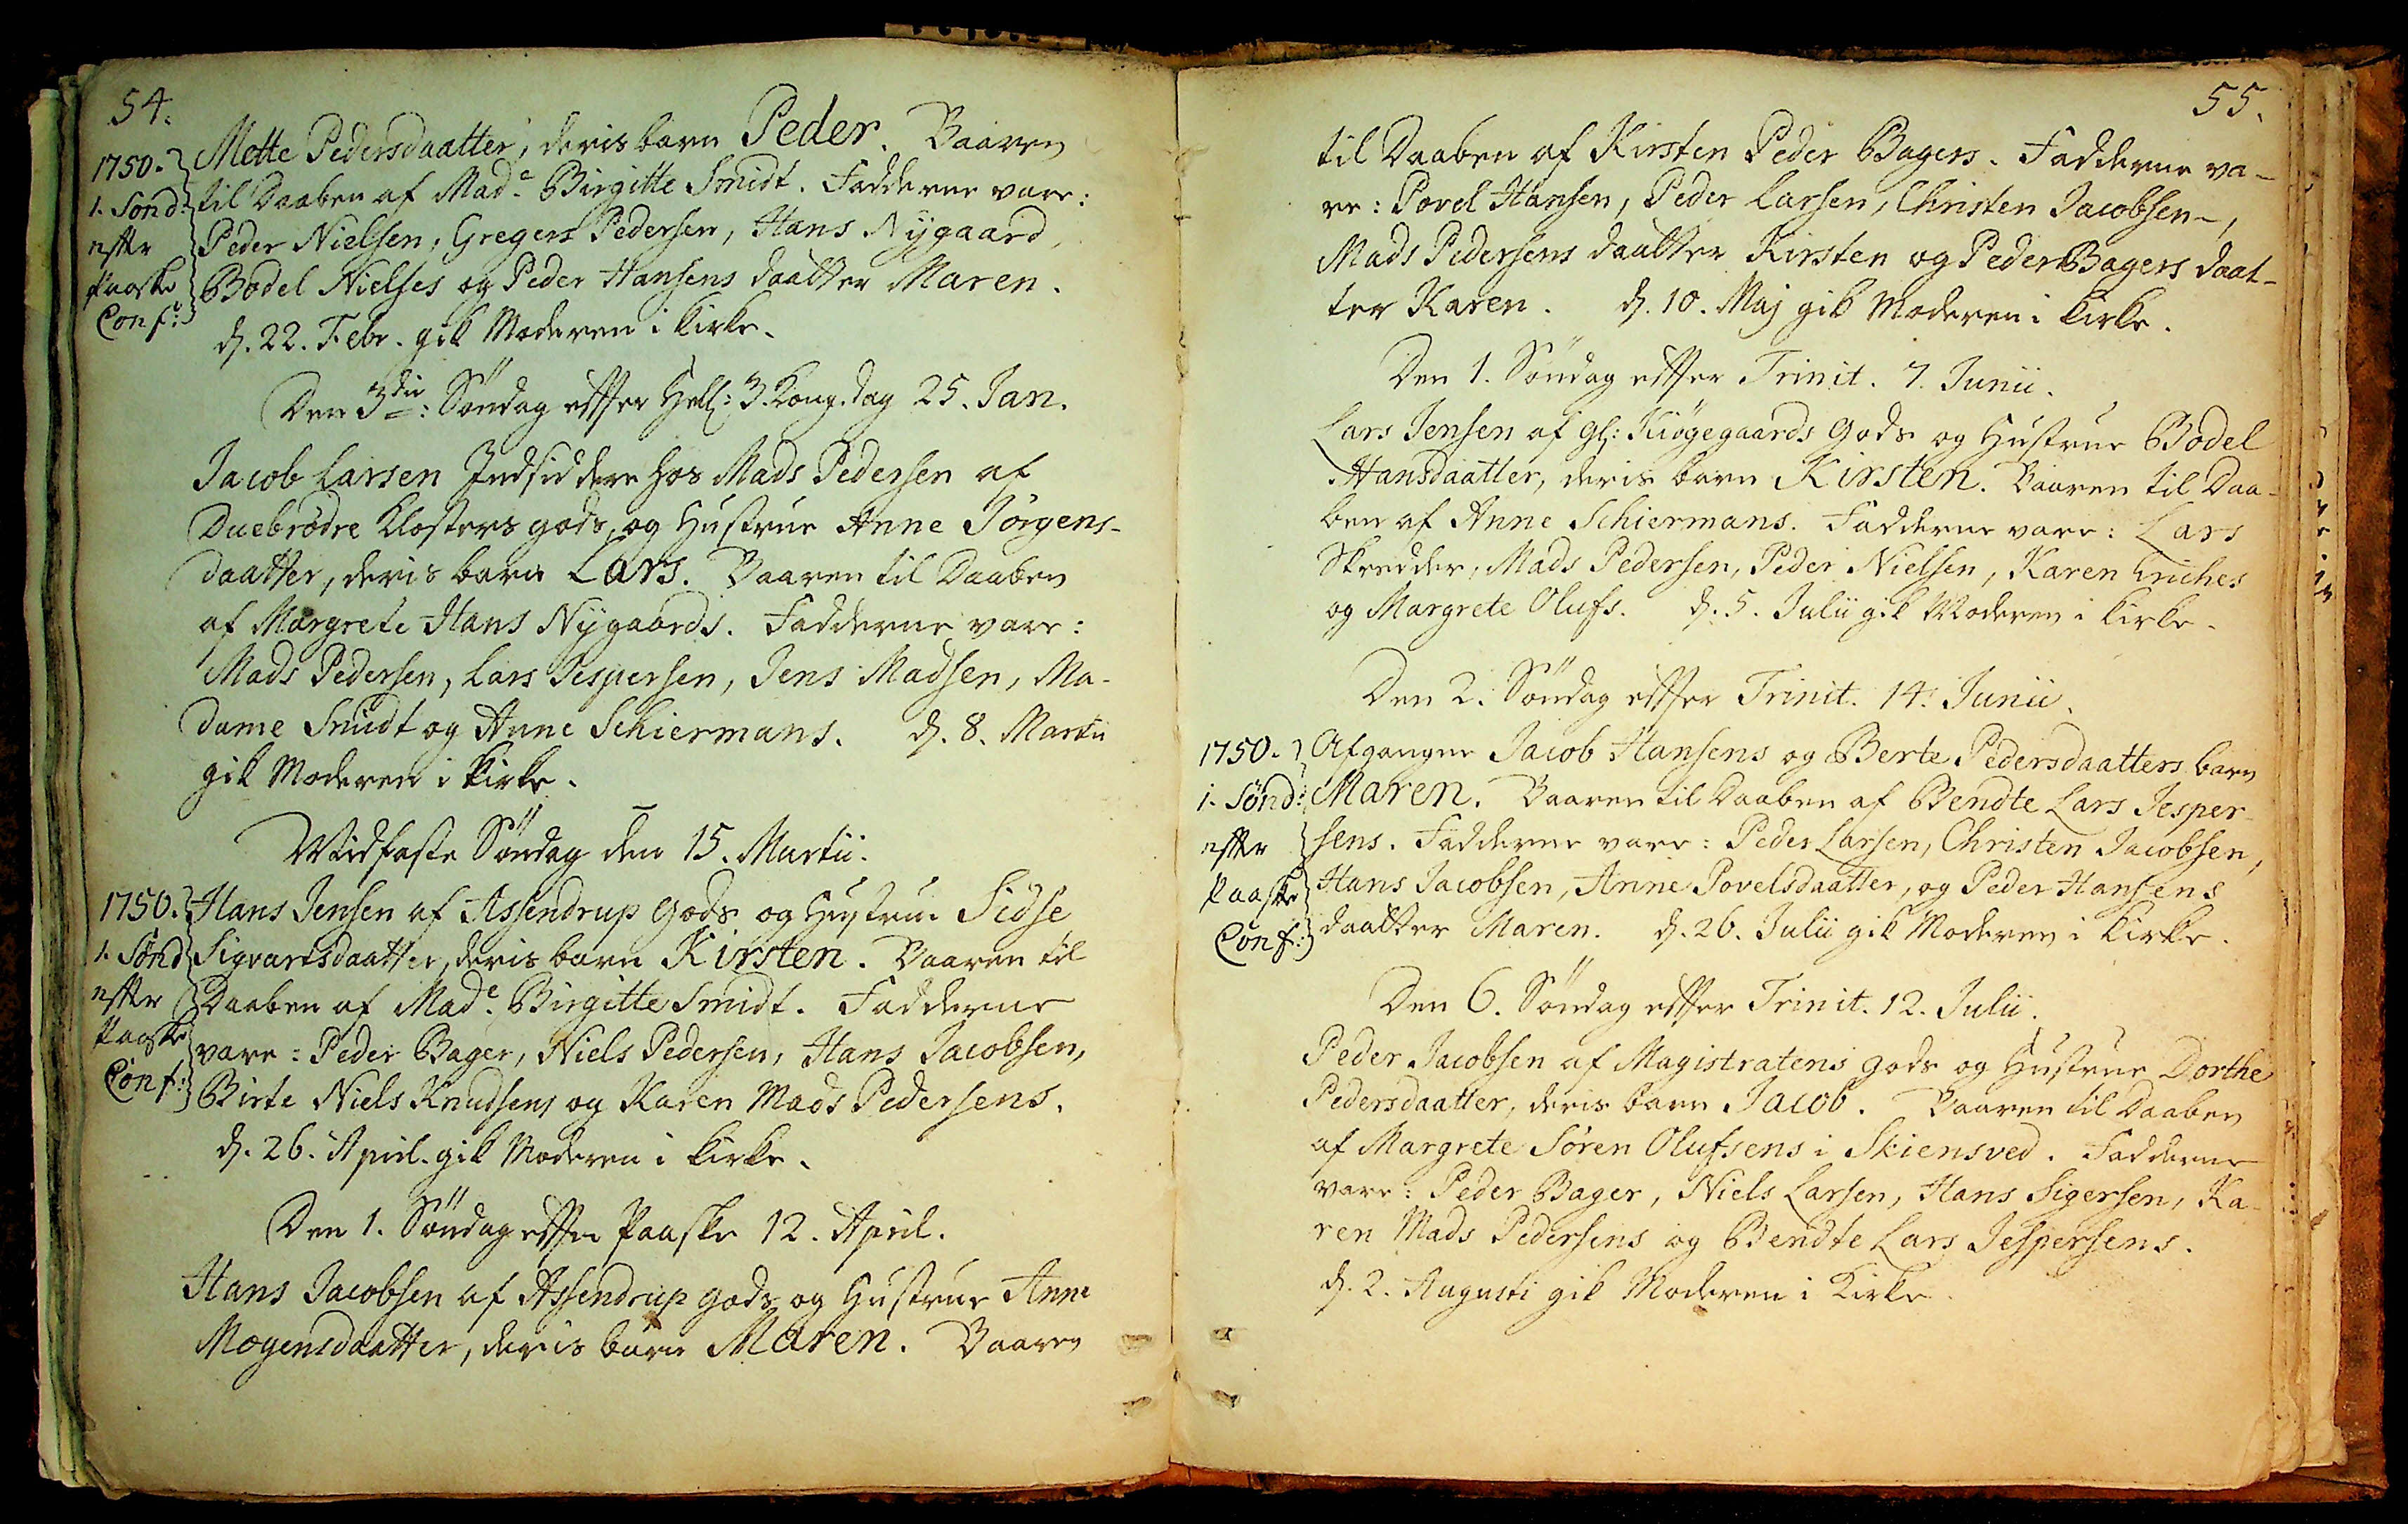54.
1750
1. Sønd:
efter
Paaske
Conf:
Mette Pedersdaatter, deris barn Peder. Baaren
til Daaben af Made: Birgitte Smidt. Fadderne vare:
Peder Nielsen, Gregers Pedersen, Hans Nygaard,
Bodel Nielses og Peder Hansens daatter Maren.
d. 22. Febr. gik Moderen i Kirke.
Den 3die: Søndag efter Hell: 3 Kong. dag 25. Jan.
Jacob Larsen Indsidder hos Mads Pedersen af
Duebrødre Klosters gods, og Hustrue Anne Jørgens
daatter, deris barn Lars. Baaren til Daaben
af Margrete Hans Nygaards. Fadderne vare:
Mads Pedersen, Lars Jespersen, Jens Madsen, Ma¬
dame Smidt og Anne Schiermans. d. 8. Martii
gik Moderen i kirke.
Midfaste Søndag den 15. Martii.
1750.
1. Sønd:
efter
Paaske
Conf:
Hans Jensen af Assendrup gods og Hustrue Sidse
Sigvartsdaatter, deris barn Kirsten. Baaren til
Daaben af Made: Birgitte Smidt. Fadderne
vare: Peder Bager, Niels Pedersen, Hans Jacobsen,
Birte Niels Knudsens og Karen Mads Pedersens.
d. 26. April gik Moderen i Kirke.
Den 1. Søndag efter Paaske 12. April.
Hans Jacobsen af Assendrup gods og Hustrue Anne
Mogensdaatter, deris barn Maren. Baaren 
55.
til Daaben af Kirsten Peder Bagers. Fadderne va¬
re: Povel Hansen, Peder Larsen, Christen Jacobsen,
Mads Pedersens daater Kirsten og Peder Bagers daat¬
ter Karen. d. 10. Maj gik Moderen i Kirke.
Den 1. Søndag efter Trinit. 7. Junii.
Lars Jensen af gl: Kiøgegaards Gods og Hustrue Bodel
Hansdaatter, deris barn Kirsten. Baaren til Daa¬
ben af Anne Schiermans. Fadderne vare: Lars
Skredder, Mads Pedersen, Peder Nielsen, Karen Eriches
og Margrete Olufs. d. 5. Julii gik Moderen i Kirke.
Den 2. Søndag efter Trinit. 14. Junii.
1750
1. Sønd:
efter
Paaske 
Conf: 
Afgangne Jacob Hansens og Berte Pedersdaatters barn
Maren. Baaren til Daaben af Bendte Lars Jesper¬
sens. Fadderne vare: Peder Larsen, Christen Jacobsen,
Hans Jacobsen, Anne Povelsdaatter, og Peder Hansens
daatter Maren. d. 26. Julii gik Moderen i Kirke.
Den 6. Søndag efter Trinit. 12. Julii.
Peder Jacobsen af Magistratens gods og Hustrue Dorthe
Pedersdaatter, deris barn Jacob. Baaren til Daaben
af Margrete Søren Olufsens i Skiensved. Fadderne
vare: Peder Bager, Niels Larsen, Hans Sigersen, Ka¬
ren Mads Pedersens og Bendte Lars Jespersens.
d. 2. Augustii gik Moderen i Kirke 
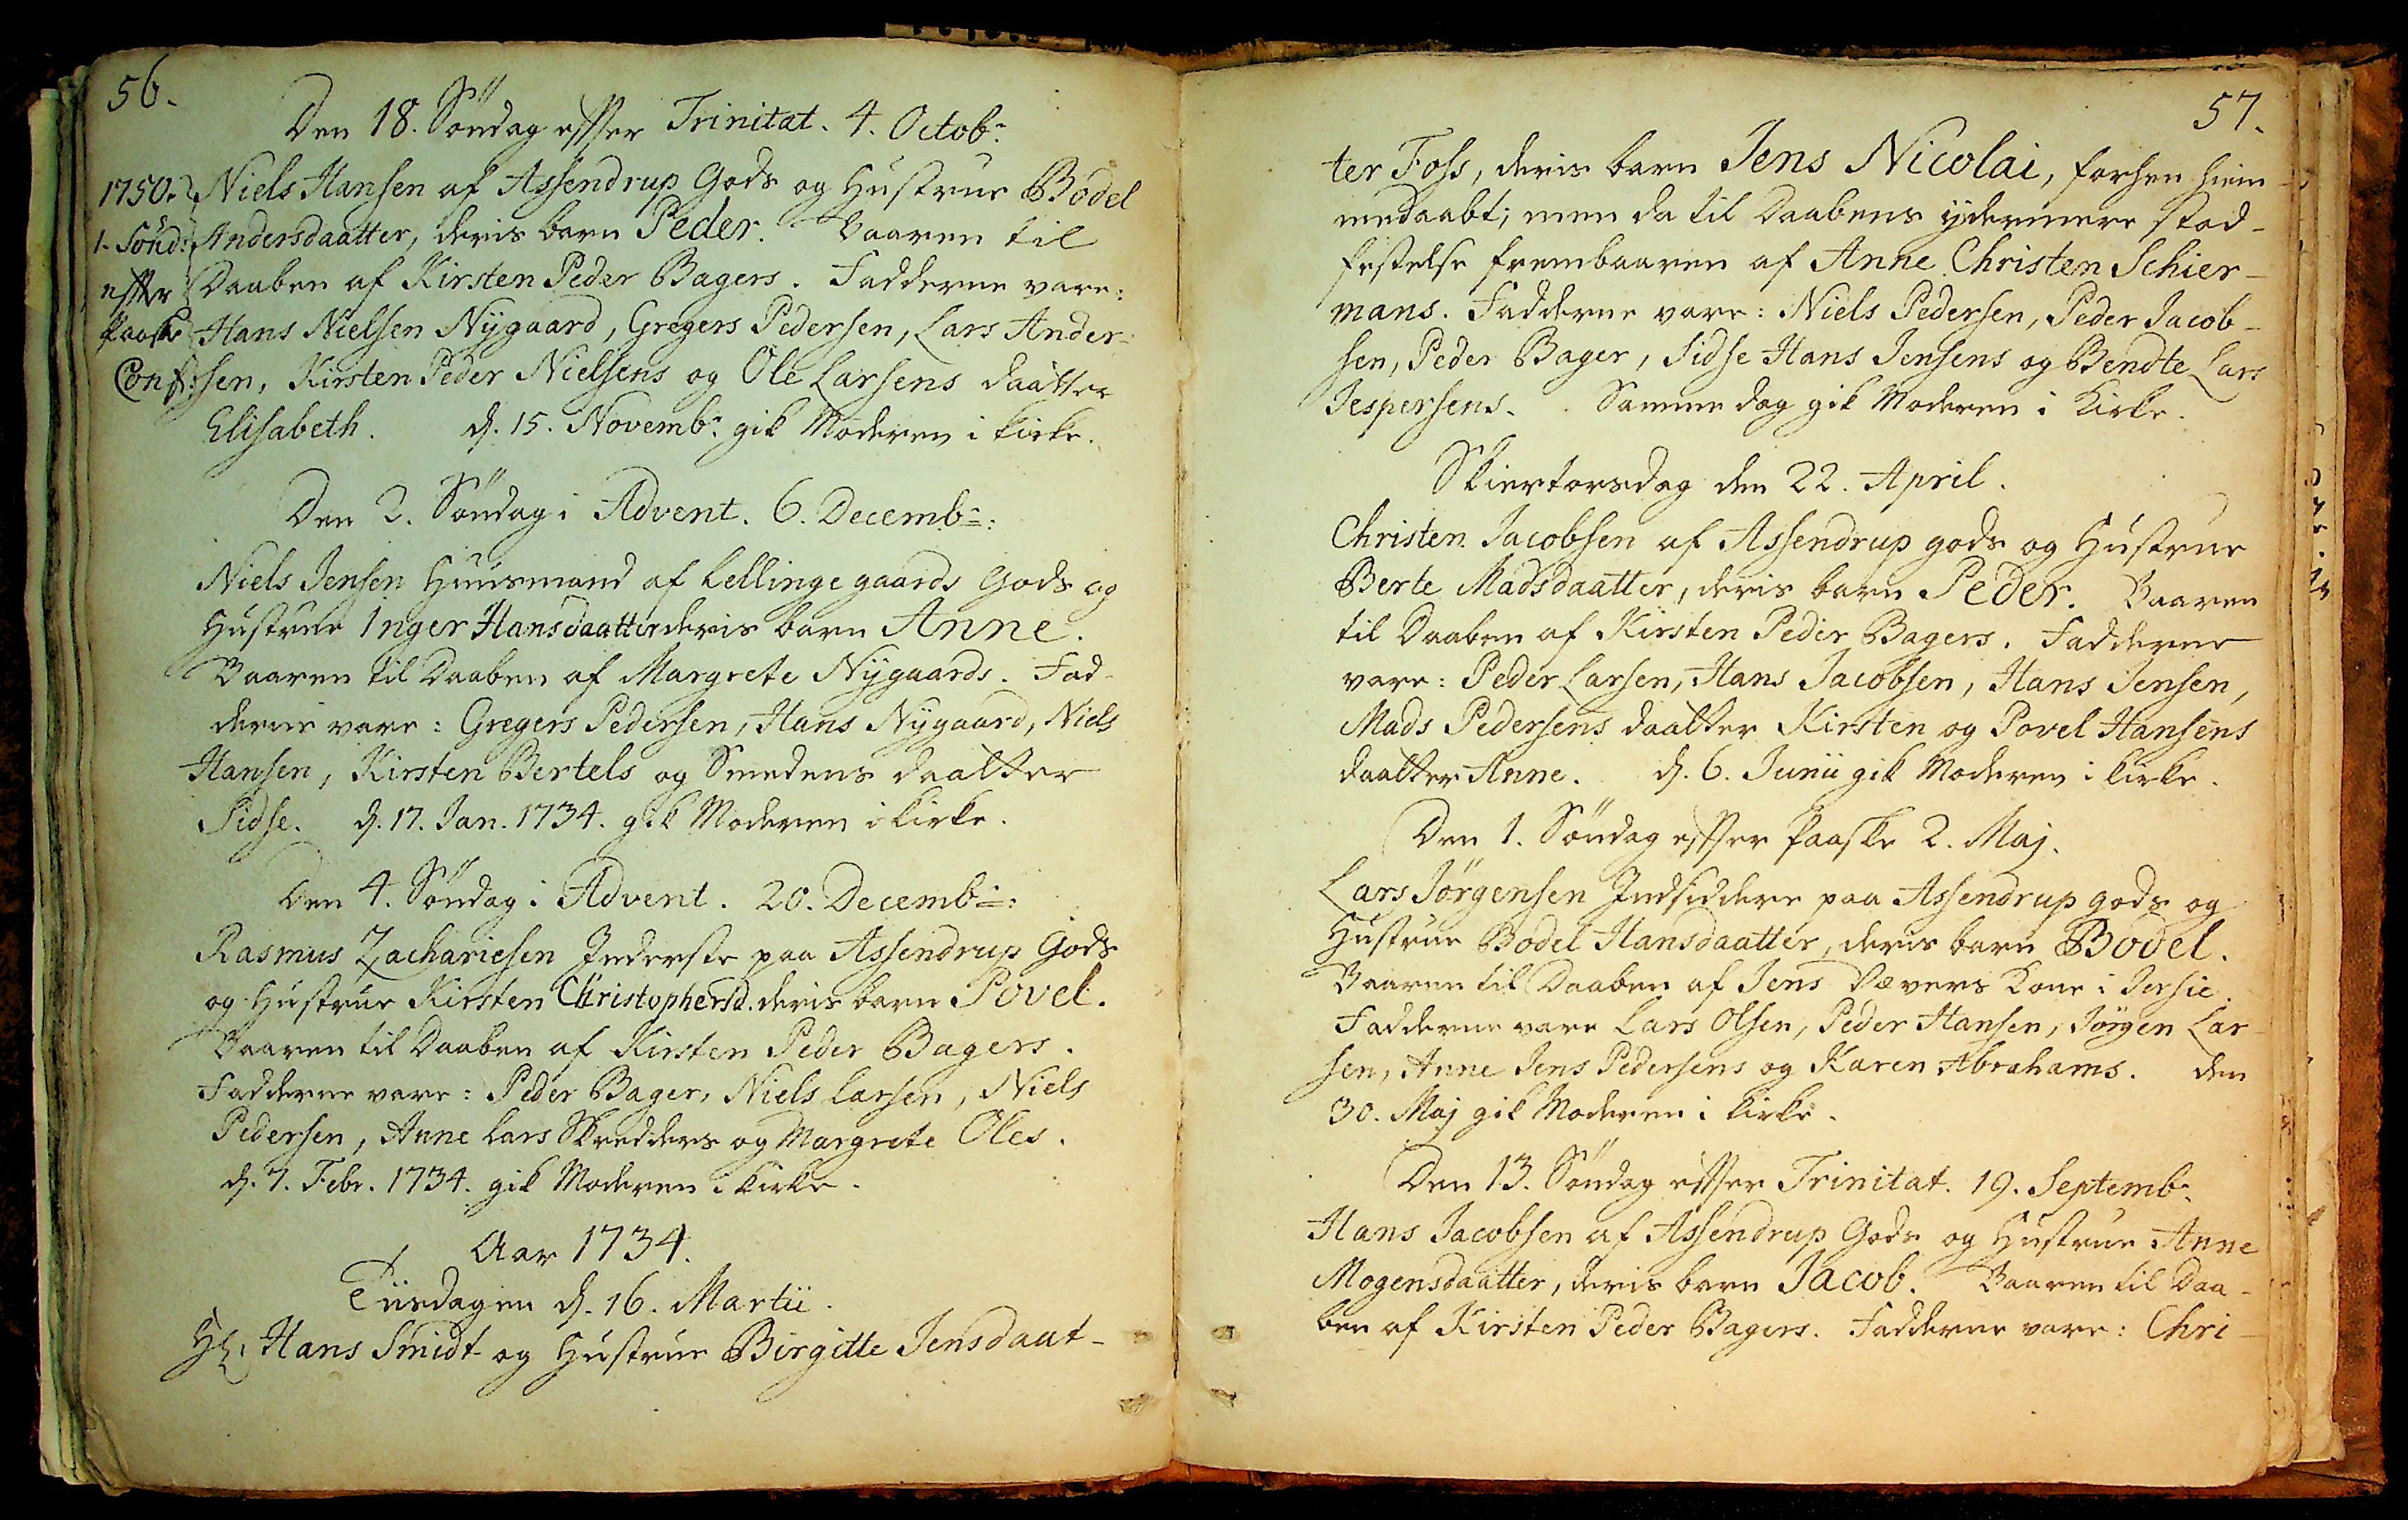56.
Den 18. Søndag efter Trinitat. 4. Octobr
1750.
1. Sønd:
efter
Paaske
Conf:
Niels Hansen af Assendrup Gods og Hustrue Bodel
Andersdaatter, deris barn Peder. Baaren til
Daaben af Kirsten Peder Bagers. Fadderne vare:
Hans Nielsen Nygaard, Gregers Pedersen, Lars Ander¬
sen, Kirsten Peder Nielsens og Ole Larsens Daatter
Elisabeth. d. 15. Novembr gik Moderen i Kirke.
Den 2. Søndag i Advent. 6. Decembr:
Niels Jensen Huusmand af Lellingegaards Gods og
Hustrue Inger Hansdaatter deris barn Anne.
Baaren til Daaben af Margrete Nygaards. Fad
derne vare: Gregers Pedersen, Hans Nygaard, Niels
Hansen, Kirsten Bertels og Smedens daatter
Sidse. d. 17. Jan. 1734. gik Moderen i Kirke.
Den 4. Søndag i Advent. 20. Decembr:
Rasmus Zachariesen Inderste paa Assendrup Gods
og Hustrue Kirsten Christophersd. deris barn Povel.
Baaren til Daaben af Kirsten Peder Bagers.
Fadderne vare: Peder Bager, Niels Larsen, Niels
Pedersen, Anne Lars Skredders og Margrete Oles.
d. 7. Febr. 1734. gik Moderen i Kirke.
Aar 1734.
Tiirsdagen d. 16. Martii.
Gl: Hans Smidt og Hustrue Birgitte Jensdaat¬
57.
ter Foss, deris barn Jens Nicolai, forhen hiem
medaabt; men da til Daabens ydermere stad¬
festelse frembaaren af Anne Christen Schier¬
mans. Fadderne vare: Niels Pedersen, Peder Jacob¬
sen, Peder Bager, Sidse Hans Jensens og Bendte Lars
Jespersens. Samme dag gik Moderen i Kirke.
Skiertorsdag den 22. April.
Christen Jacobsen af Assendrup Gods og Hustrue
Berte Madsdaatter, deries barn Peder. Baaren
til Daaben af Kirsten Peder Bagers. Fadderne
vare: Peder Larsen, Hans Jacobsen, Hans Jensen,
Mads Pedersens daatter Kirsten og Povel Hansens
daatter Anne. d. 6. Junii gik Moderen i Kirke.
Den 1. Søndag efter Paaske 2. Maj.
Lars Jørgensen Indsiddere paa Assendrup gods og
Hustrue Bodel Hansdaatter, deris barn Bodel.
Baaren til Daaben af Jens Vævers Kone i Jersie.
Fadderne vare Lars Olsen, Peder Hansen, Jørgen Lar¬
sen, Anne Jens Pedersens og Karen Abrahams. den
30. Maj gik Moderen i Kirke.
Den 13. Søndag efter Trinitat. 19. Septembr
Hans Jacobsen af Assendrup Gods og Hustrue Anne
Mogensdaatter, deris barn Jacob. Baaren til Daa¬
ben af Kirsten Peder Bagers. Fadderne vare: Chri¬